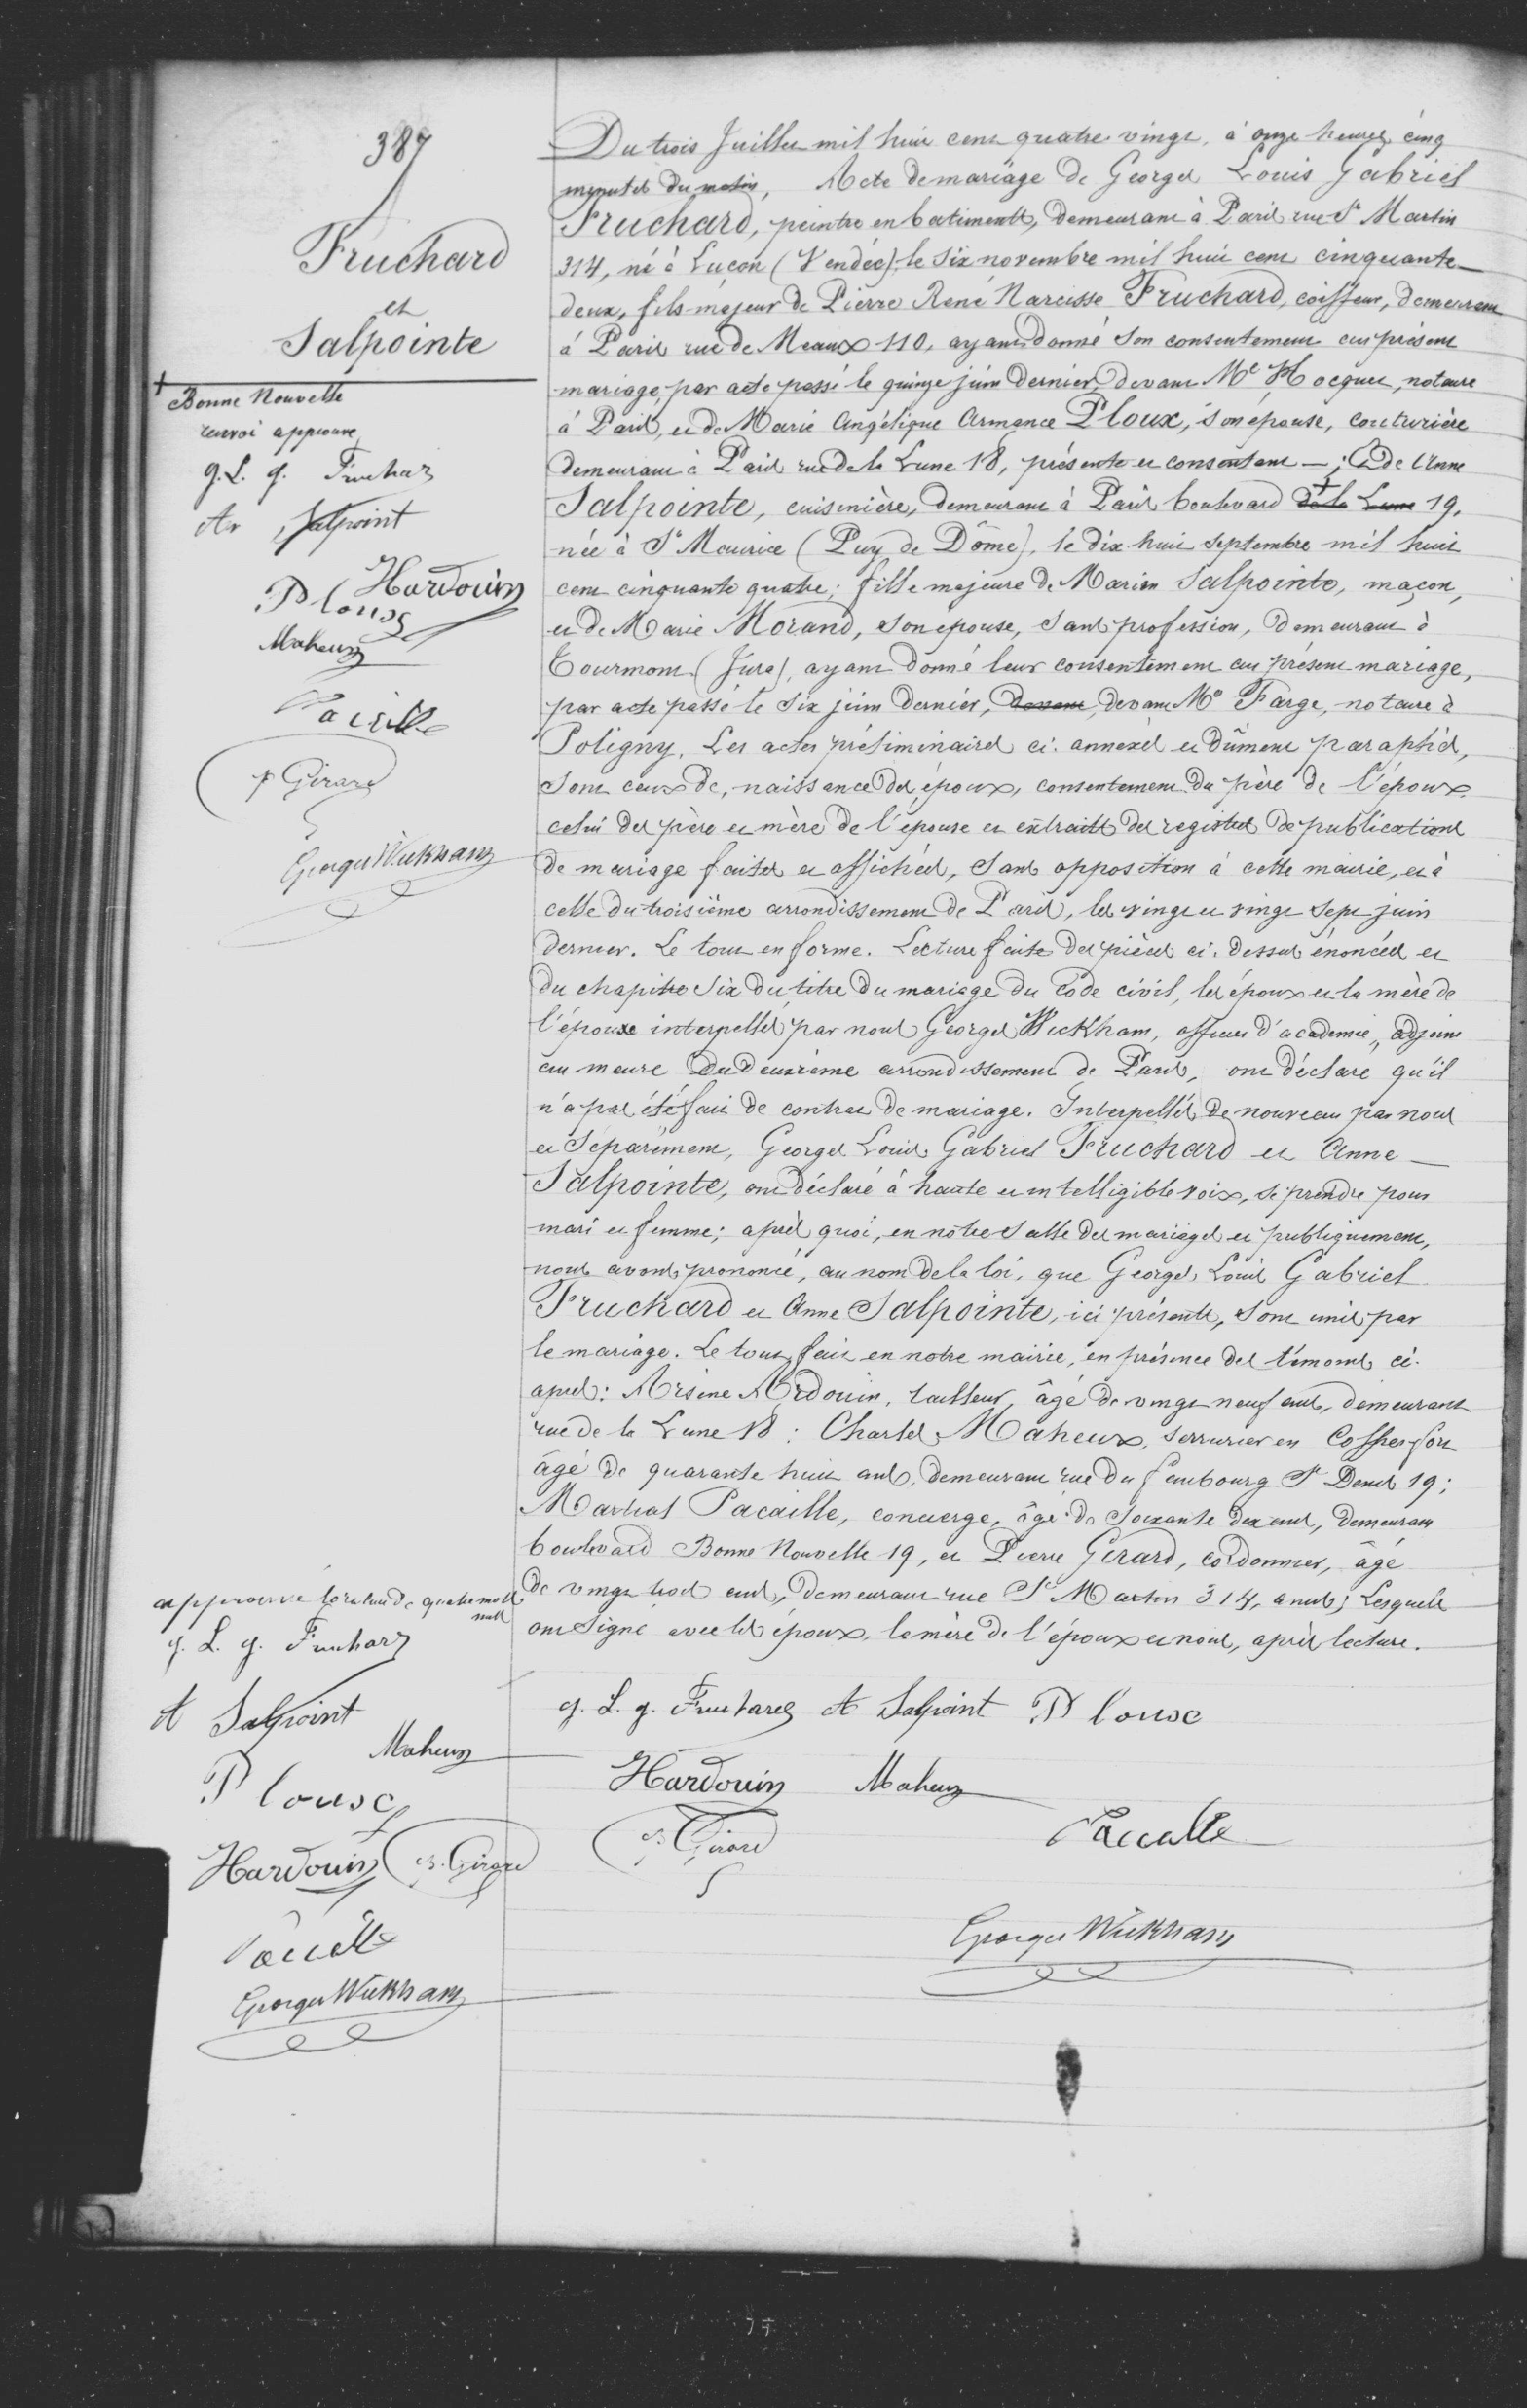387
Fruchard
et
Salpointe
Du trois Juillet mil huit cent quatre vingt, à onze heures cinq
minutes du matin, Acte de mariage de Georges Louis Gabriel
Fruchard, peintre en batiment, demeurant à Paris, rue St Martin
314, né à Luçon (Vendée) le six novembre mil huit cent cinquante-
deux, fils majeur de Pierre René Narcisse Fruchard, coiffeur, demeurant
à Paris rue de Meaux 110, ayant donné son consentement en présence
mariage par acte passé le quinze juin dernier, devant M° Hocques, notaire
à Paris, et de Marie Angélique Armance Ploux, son épouse, couturière
demeurant à Paris rue de la Lune 18, présente et consentante -; De Anne
Salpointe, cuisinière, demeurant à Paris boulevard de la Lune 19,
née à St Maurice (Puy de Dôme), le dix huit septembre mil huit
cent cinquante quatre; fille majeur de Marion Salpointe, maçon,
et de Marie Morand, son epouse, sans profession, demeurant à
Tourmont (Jura) ayant donné leur consentement au présent mariage,
par acte passé le dix juin dernier, devant devant Me Farge, notaire à
Poligny, Les actes préliminaires ci-annexés et dûment paraphés
sont ceux de, naissance des époux, consentement du père de l'époux
celui du père et mère de l'épouse et extrait du registre de publication
de mariage faiset et affichés, sans opposition à cette mairie, et à
celle du troisième arrondissement de Paris, les vingt et vingt sept juin
dernier. Le tout en forme. Lecture faite des pièces ci-dessus énoncées
du chapitre Six du titre du mariage du code civil, les époux et la mère de
l'épouse interpellé par nous Georges Wickham, officier d'académie, adjoint
au maire, du deuxième arrondissement de Paris, ont déclaré qu'il
n'a pas été fait de contrat de mariage. Interpellés de nouveau par nous
et séparément, Georges Louis Gabriel Fruchard et Anne
Salpointe, ont déclaré à haute et intelligible voix, se prendre pour
mari et femme; après quoi, en notre salle du mariage et publiquement,
nous avons prononcé au nom de la loi, que Georges, Louis Gabriel
Fruchard et Anne Salpointe, ici présents, sont unis par
le mariage. Le tous fais en notre mairie, en présence des témoins ci
après: Arsène Ardoins, tailleur âgé de vingt neuf ans, demeurant
rue de la Lune 18: Charles Maheux, serrurier en Coffres-fort
âgé de quarante huit ans, demeurant rue du faubourg St Denis 19;
Martras Pacaille, concierge, âgé de soixante dix ans, demeurant
boulevard Bonne Nouvelle 19, et Pierre Gerard, cordonnier, âgé
de vingt trois ans, demeurant rue St Martin 314, amis, Lesquels
ont signé avec les époux, la mère de l'époux et nous, après lecture.
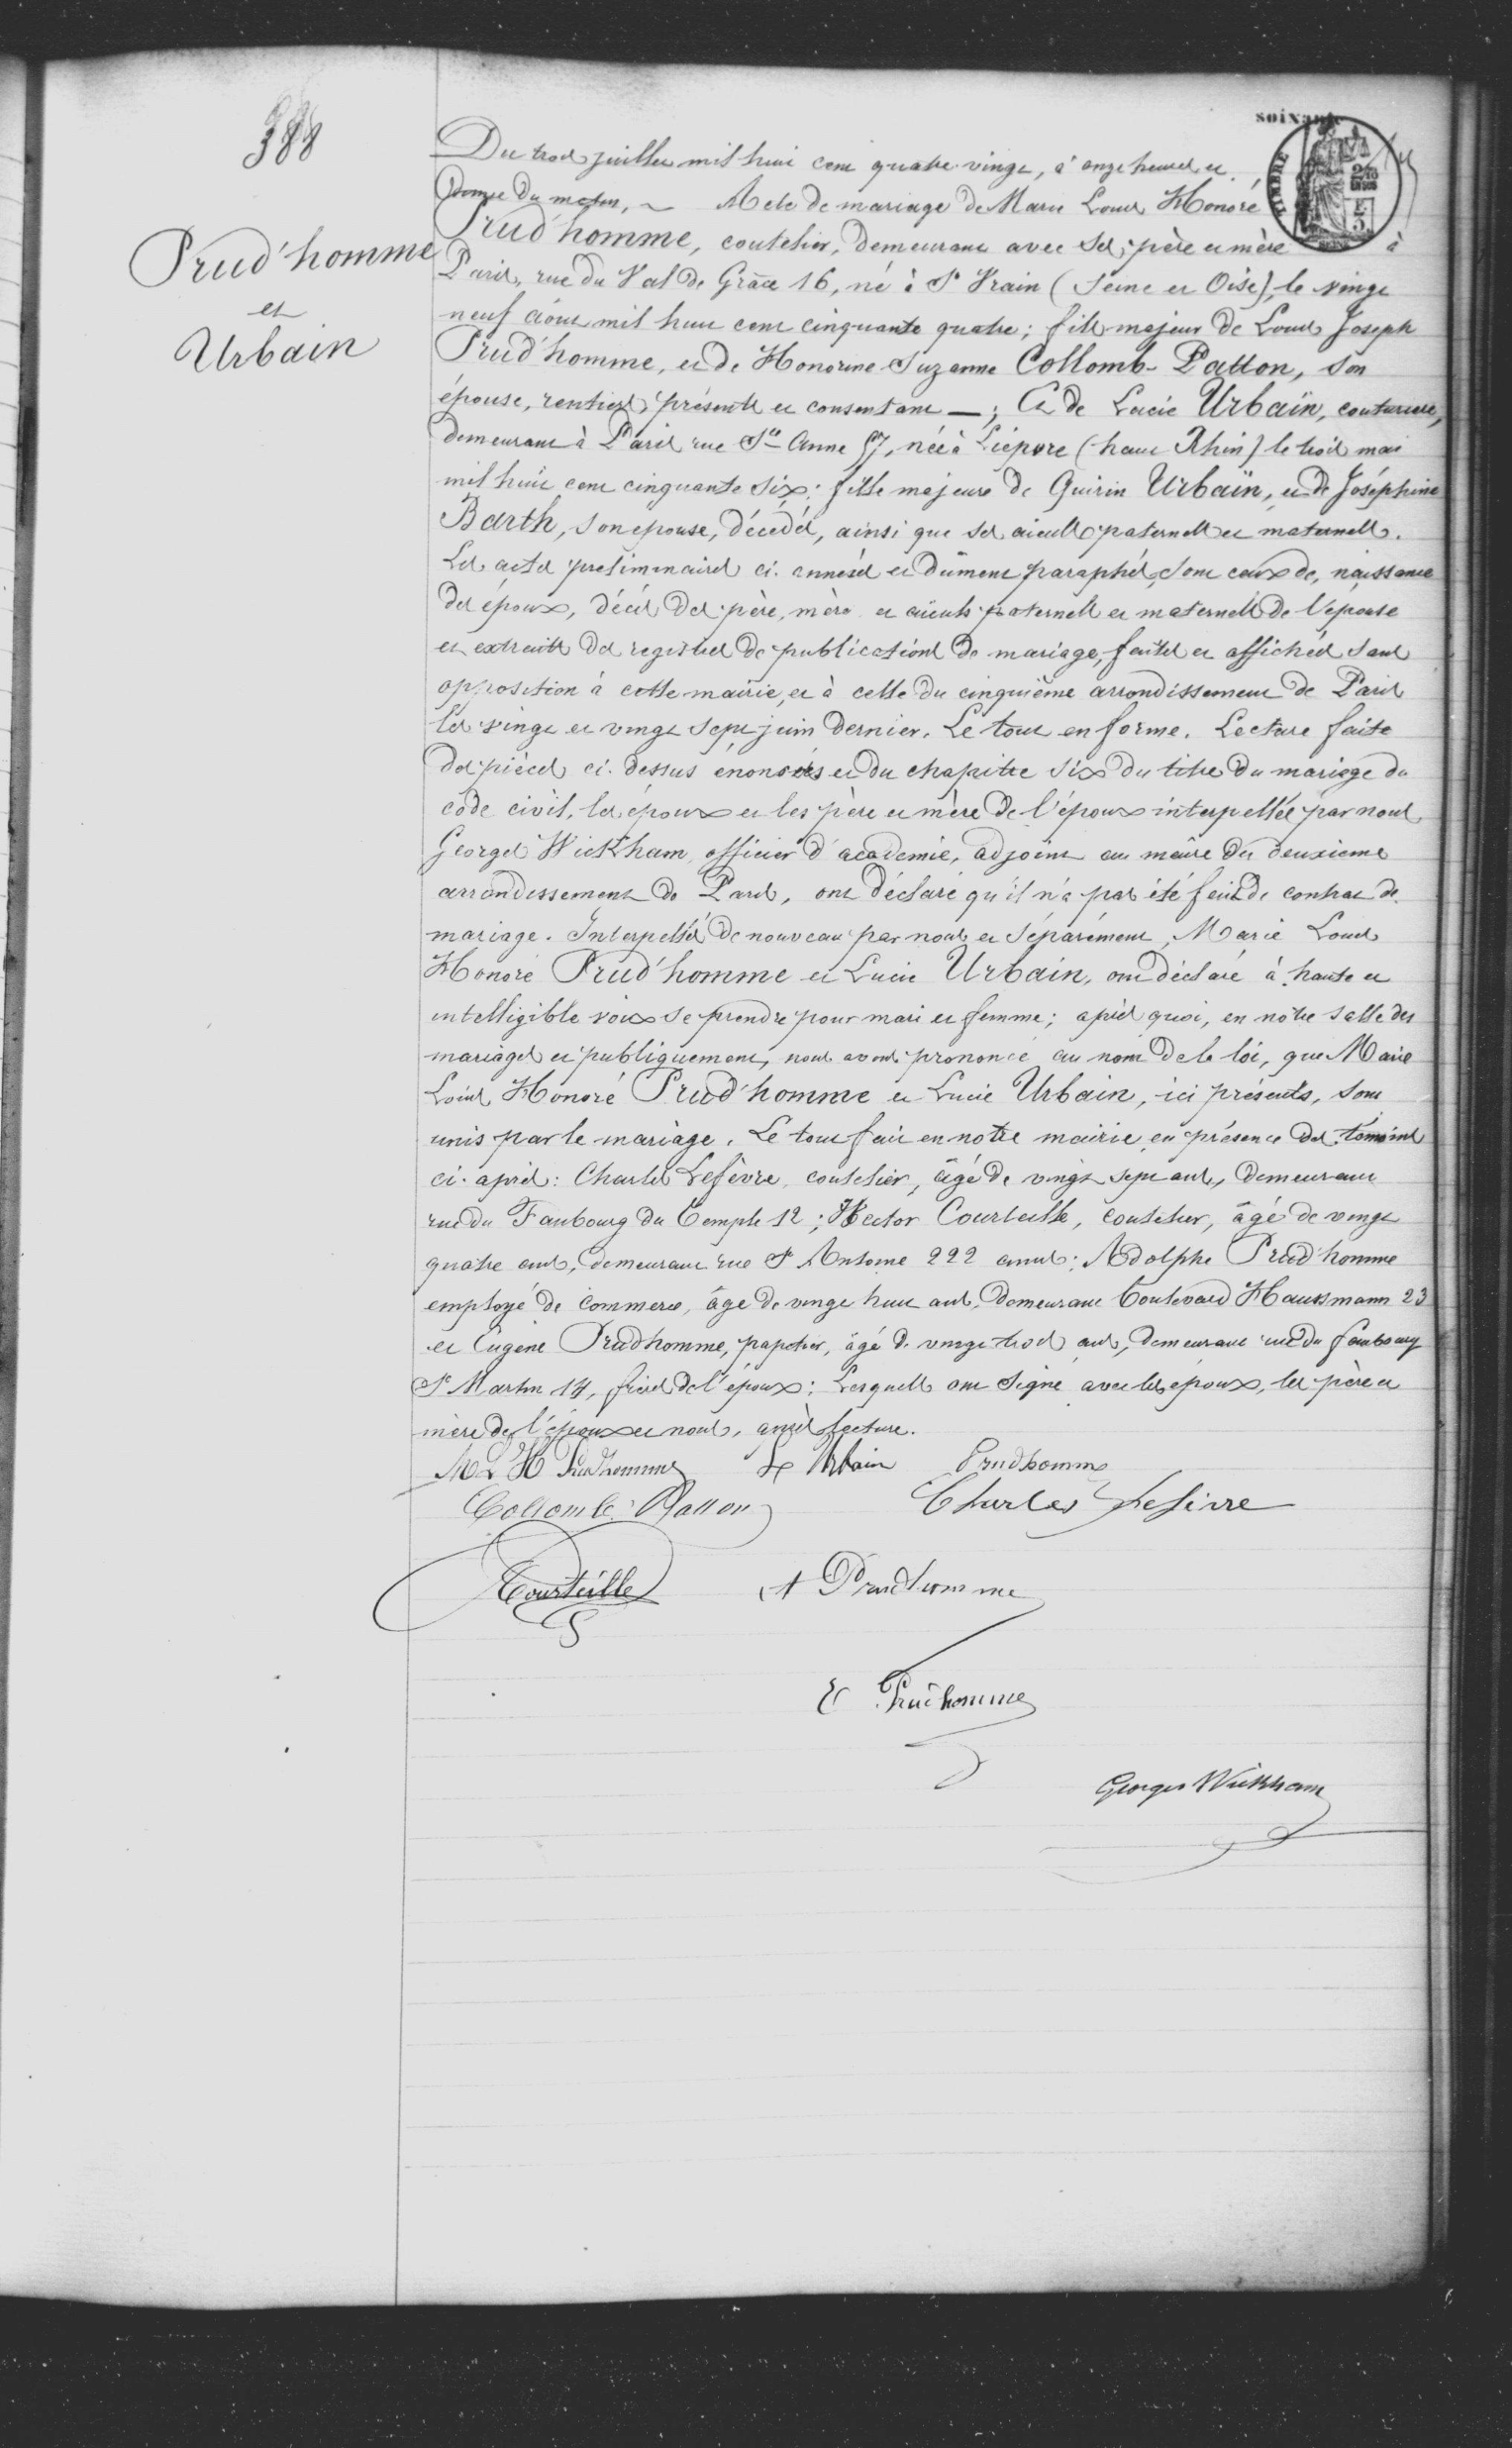388
Prud'homme
et
Urbain
Du trois juillet mil huit cent quatre-vingt, à onze heures
Douze du matin -Acte de mariage de Marie Louis Honoré
Prud'homme, coutelier, demeurant avec ses père et mère à
Paris, rue du Val de Grâce 16, né à St Yrain (Seine et Oise) le vingt
neuf aout mil huit cent cinquante quatre; fils majeur de Louis Joseph
Prud'homme, et de Honorine Suzanne Colomb Patton, son
épouse, rentiers présente et consentant-; Ce de Lucie Urbain, couturiere,
demeurant à Paris rue Ste Anne 57, née à Liépore (haut Rhin) le trois mai
mil huit cent cinquante six, fille majeure de Quirin Urbain, et de Joséphine
Barth, son épouse, décédée, ainsi que ses aieuls paternels et maternels.
Les actes préliminaires ci-annexés et dûment paraphés donc ceux de naissance
des époux, décès des père, mère, et aïeuls paternels en maternels de l'épouse
et extraite de registier de publications de mariages, faites en affiche sans
opposition à cette mairie, et à celle du cinquième arrondissement de Paris
les vingt et vingt sept juin dernier. Le tous en forme. Lecture faite
des pièces ci-dessus énoncés du chapitre six du titre du mariage du
code civil, les époux et les père et mère de l'époux interpellée par nous
George Wickmam, officier d'académie, adjoint au maire du deuxieme
arrondissement de Paris, ont déclaré qu'il n'a pas été fait de contrat de
mariage. Interpellés de nouveau pas nous et séparément Marie Louis
Honoré Prud'homme et Lucie Urbain, ont déclaré à haute et
intelligible voix se prendre pour mari et femme; après quoi, en notre salle de
mariage et publiquement, nous avons prononcé au nom de la loi, que Marie
Louis Honoré Prud'homme et Lucie Urbain, ici présents, son
unis par le mariage. Le tout fait en notre mairie, en présence de témoins
ci après. Charles Lefèvre, coutelier, âgé, vingt sept ans, demeurant
rue du Faubourg du Temple 12, Hector Courteille, conseteur, âge de vingt
quatre ans, demeurant rue St Antoine 222, amis: Adolphe Prud'homme
employé de commerce, âgé de vingt huit ans, demeurant boulevard Haussmann 23
et Eugène Prud'homme, papetier, âgé de vingt trois ans, demeurant rue du faubourg
St Martin 14, frère de l'époux: Lesquels ont signé avec les époux, le père et
mère de l'époux et nous, après lecture.